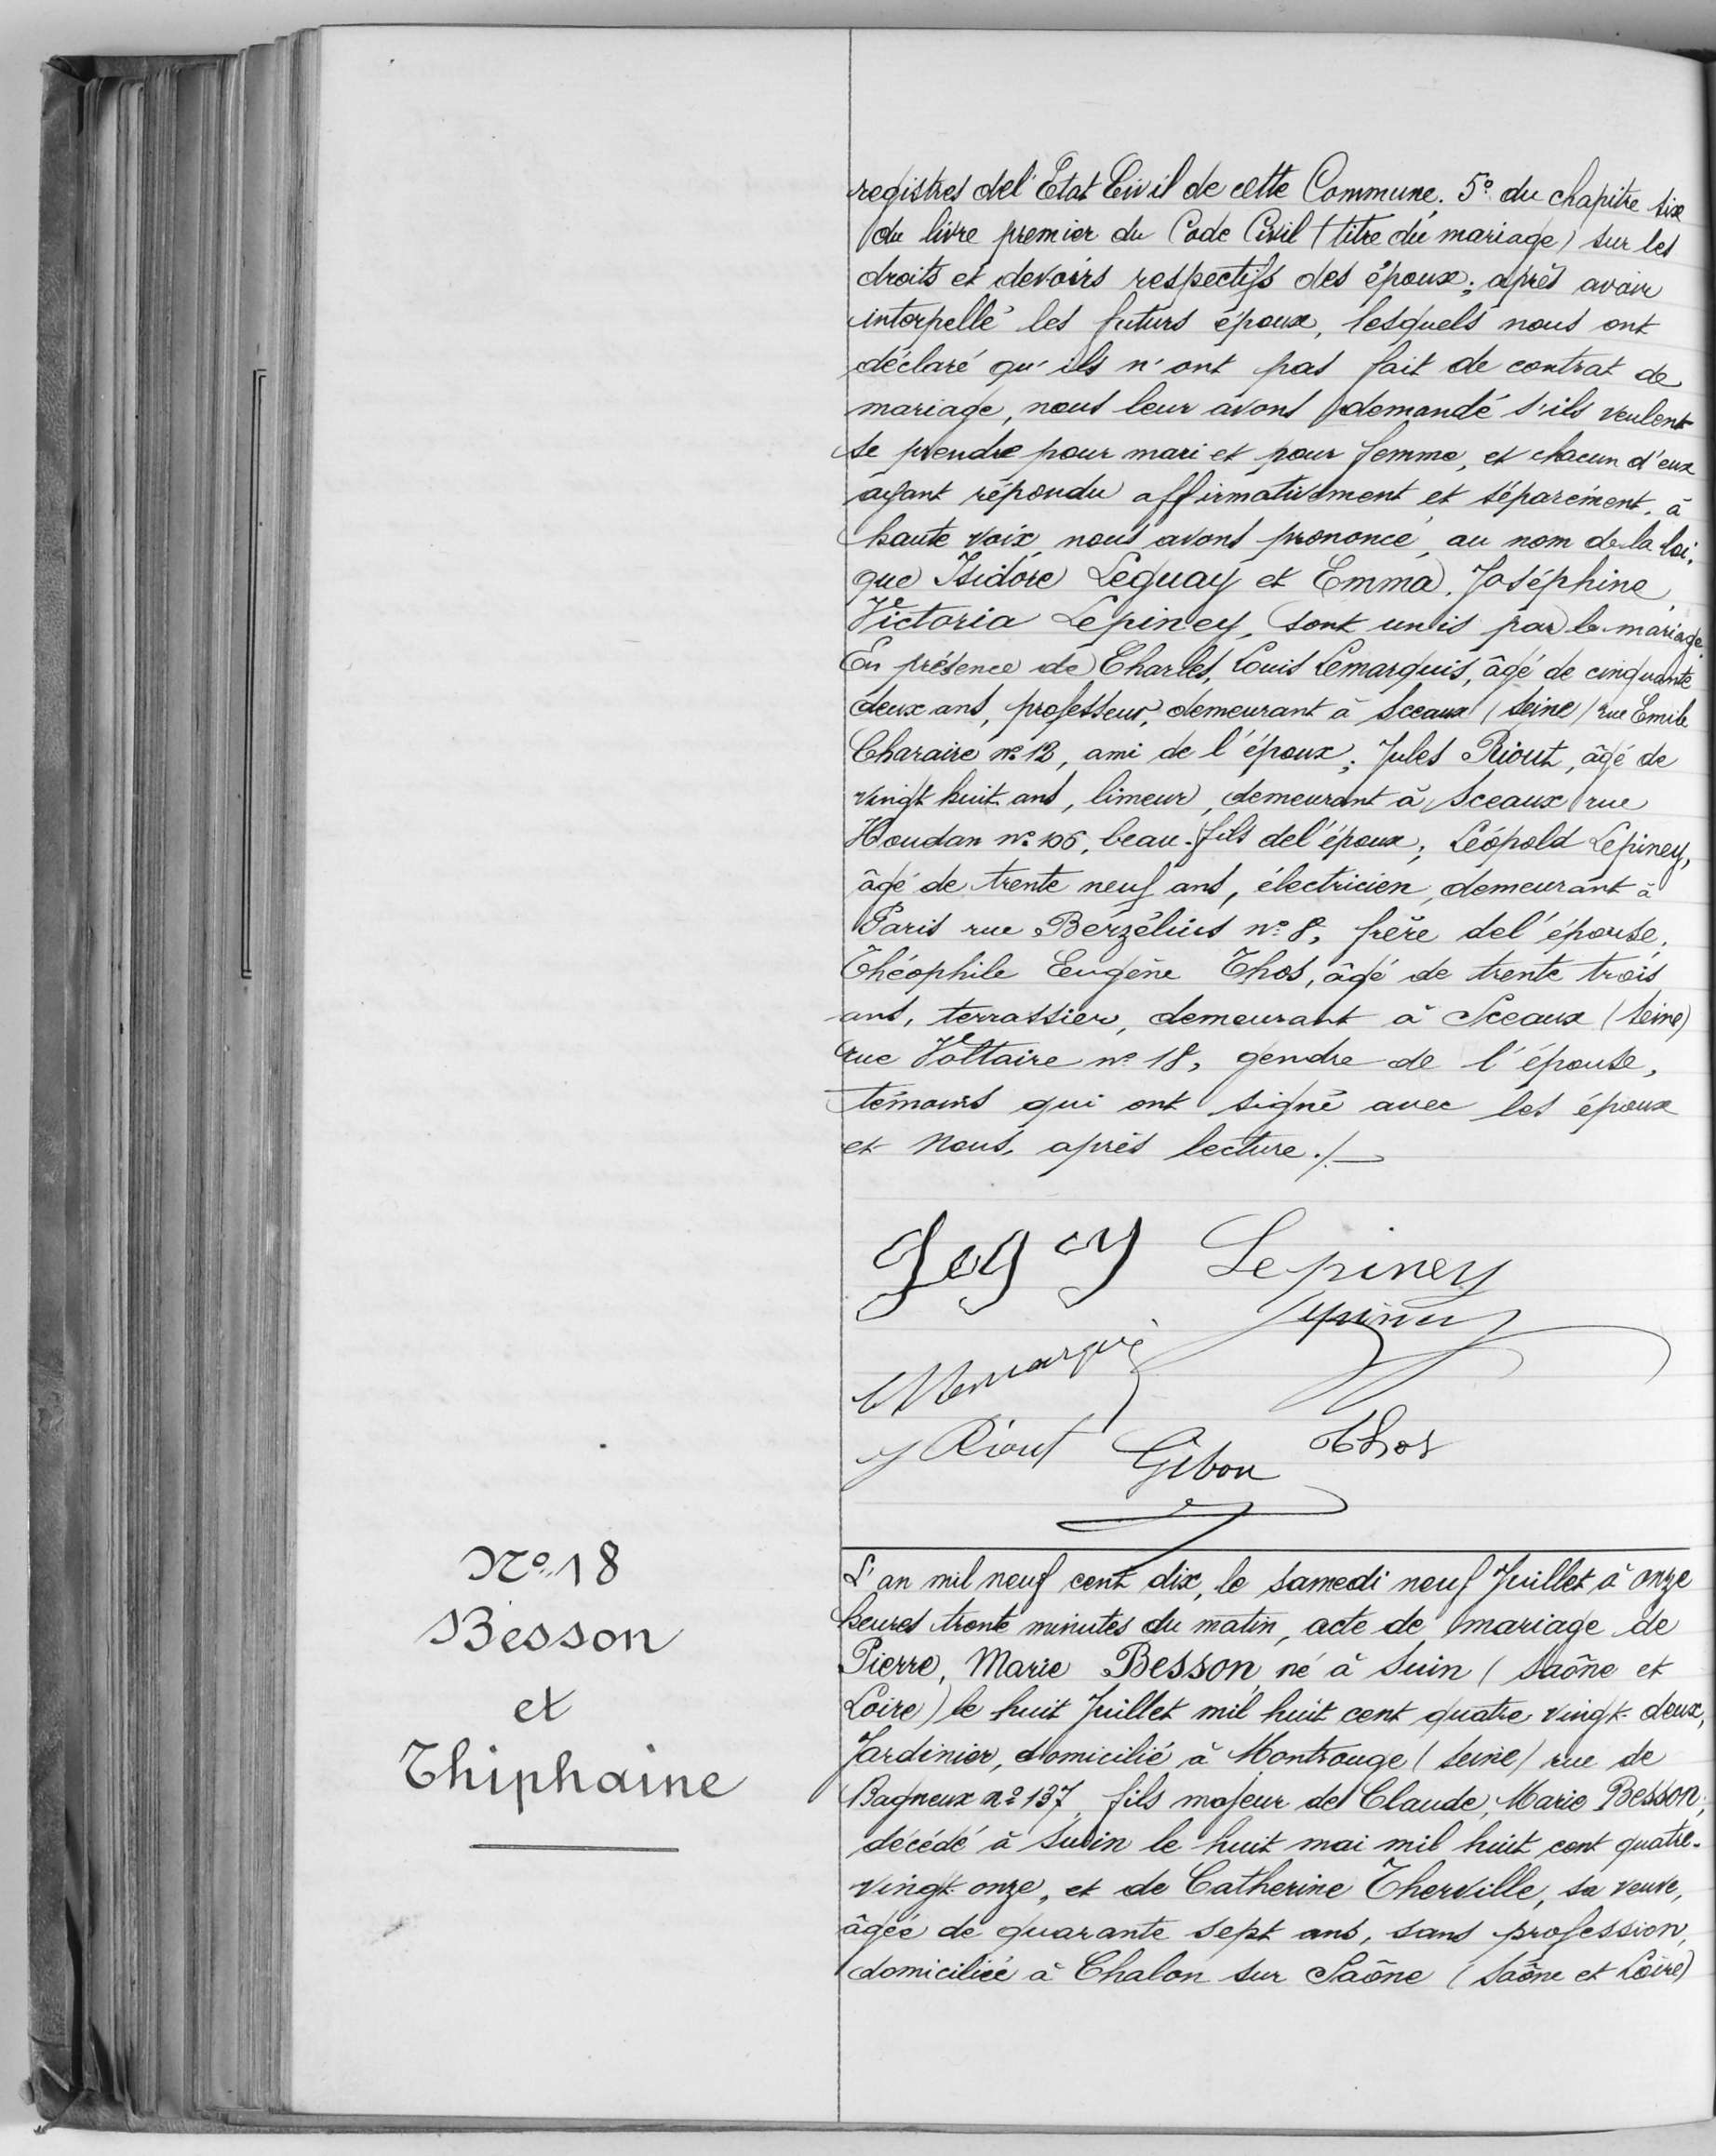registres del'Etat Civil de cette Commune. 5° du chapitre six
du livre premier du Code Civil (titre du mariage) sur les
droits et devoirs respctifs des époux, après avoir
interpellé les futurs époux, lesquels nous ont
déclaré qu'ils n'ont pas fait de contrat de
mariage, nous leur avons demandé s'ils veulent
se prendre pour mari et pour femme, et chacun d'eux
ayant répondu affirmativement et séparément, à
haute voix, nous avons prononcé, au nom de la loi,
que Isidore Lequay et Emma, Joséphine,
Victoria Lepiney, sont unies par le mariage
En présence de Charles, Louis Lemarquis, âgé de cinquante
deux ans, professeur demeurant à Sceaux (Seine) rue Emile
Charaire n°13, ami de l'époux; Jules Riout, âgé de
vingt-huit ans, limeur, demeurant à Sceaux, rue
Houdant n°106, beau-fils del'époux, Léopold Lepiney,
âgé de trente neuf ans, électricien, demeurant à
Paris rue Berzélies n°8, frère del'épouse.
Théophile Eugène Thos, âgé de trente trois
ans, terrassier, demeurant à Sceaux (Seine)
rue Voltaire n°18, gendre de l'épouse,
témoins qui ont signé avec les époux
et Nous après lecture./.
N°18
Besson
et
Thiphaine
L'an mil neuf cent dix, le samedi neuf Juillet à onze
heures trante minutes du matin, acte de mariage de
Pierre, Marie Besson, né à Suin (Saône et
Loire) le huit Juillet mil huit cent quatre vingt-deux
Jardinier, domicilié, à Montrouge (Seine-rue de
Bagneux n°137, fils majeur de Claude, Marie, Besson,
décédé à Suin le huit mai mil huit cent quatre-
vingt onze, et de Catherine Therville, sa veuve,
âgée de quarante sept-ans, sans profession
domiciliée à Chalon sur Saône (Saône et Loire)
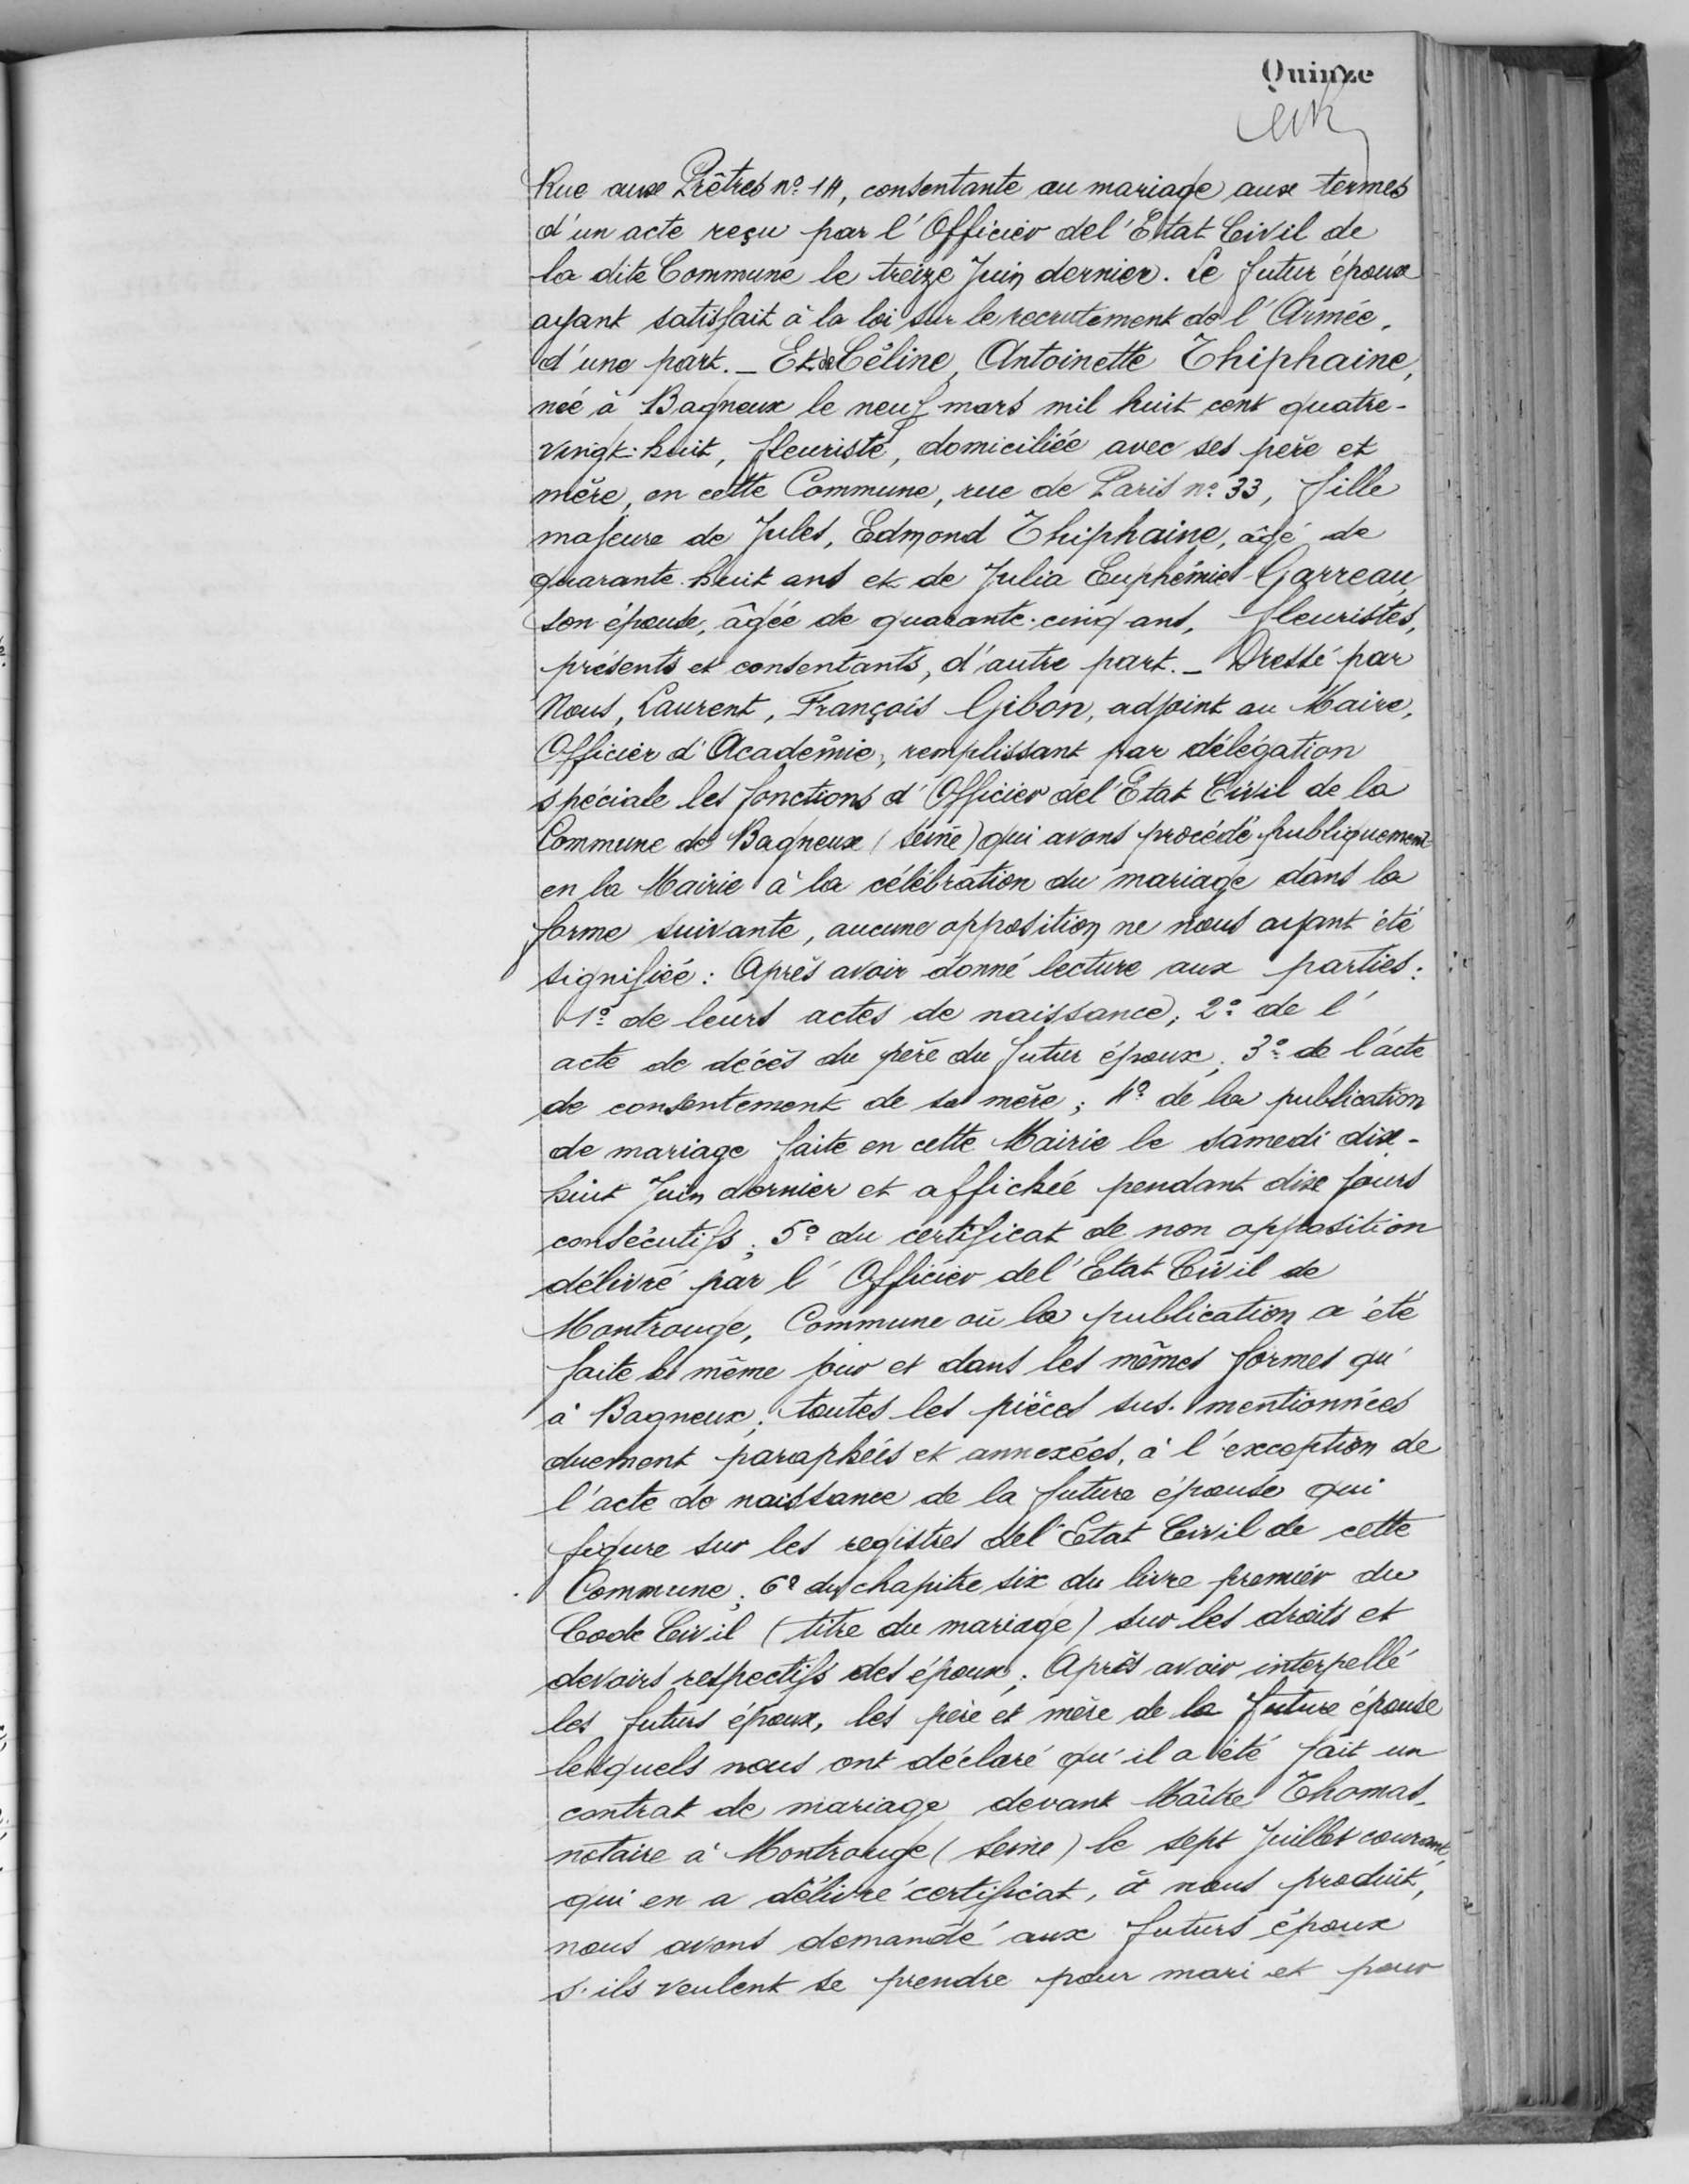Rue aux Prêtres n°14, consentante au mariage aux termes
d'un acte reçu par l'Officier del'Etat Civil de
la dite Commune le treize Juin dernier. Le futur époux
ayant satisfait à la loi sur le recrutement de l'Armée.
d'un part.-Et de Céline Antoinette Thiphaine,
née à Bagneux le neuf mars mil huit cent quatre-
vingt-huit, fleuriste, domiciliée avec ses père et
mère, en cette Commune, rue de Paris n°33, fille
majeure de Jules Edmond Thiphaine, âgé de
quarante-huit ans et de Julia Euphénie Garreau
son épouse, âgée de quarante-cinq ans, fleuristes,
présents et consentants, d'autre part.-Dressé par
Nous, Laurent, François Gibon, adjoint au Maire,
Officier d'Académie, remplissant par délégation
Spéciale les fonction d'officier del'Etat Civil de la
Commune de Bagneux (Seine) qui avons procédé publiquement
en la Mairie à la célébration du mariage dans la
forme suivante, aucune opposition ne nous ayant été
signifiée: Après avoir donné lecture aux parties:
1° de leurs actes de naissance, 2° de l'
acte de décés du père du futur époux; 3° de l'acte
de consentement de la mère; 4° de la publication
de mariage faite en cette Mairie le samedi dix-
huit Juin dernier et affichée pendant dix jours
consécutifs; 5° du certificat de non opposition
délivré par l'Officier del'Etat Civil de
Montrouge, Commune où la publication a été
faite le même jour et dans les mêmes formes qu'
à Bagneux; toutes les pièces sus mentionnées
duement paraphées et annexées, à l'exception de
l'acte de naissance de la future épouse qui
figure sur les registres del'Etat Civil de cette
Commune; 6° du chapitre six du livre premier du
Code Civil (titre du mariage) sur les droits et
devoirs respectifs del'époux; Après avoir interpellé
les futurs époux, les père et mère de la future épouse
lesquels nous ont déclaré qu'il a été fait un
contrat de mariage devant Maître Thomas,
notaire à Montrouge (Seine) le sept Juillet courant
qui en a délivré certificat, à nous produit,
nous avons demandé aux futurs époux
s'ils veulent se prendre pour mari et pour